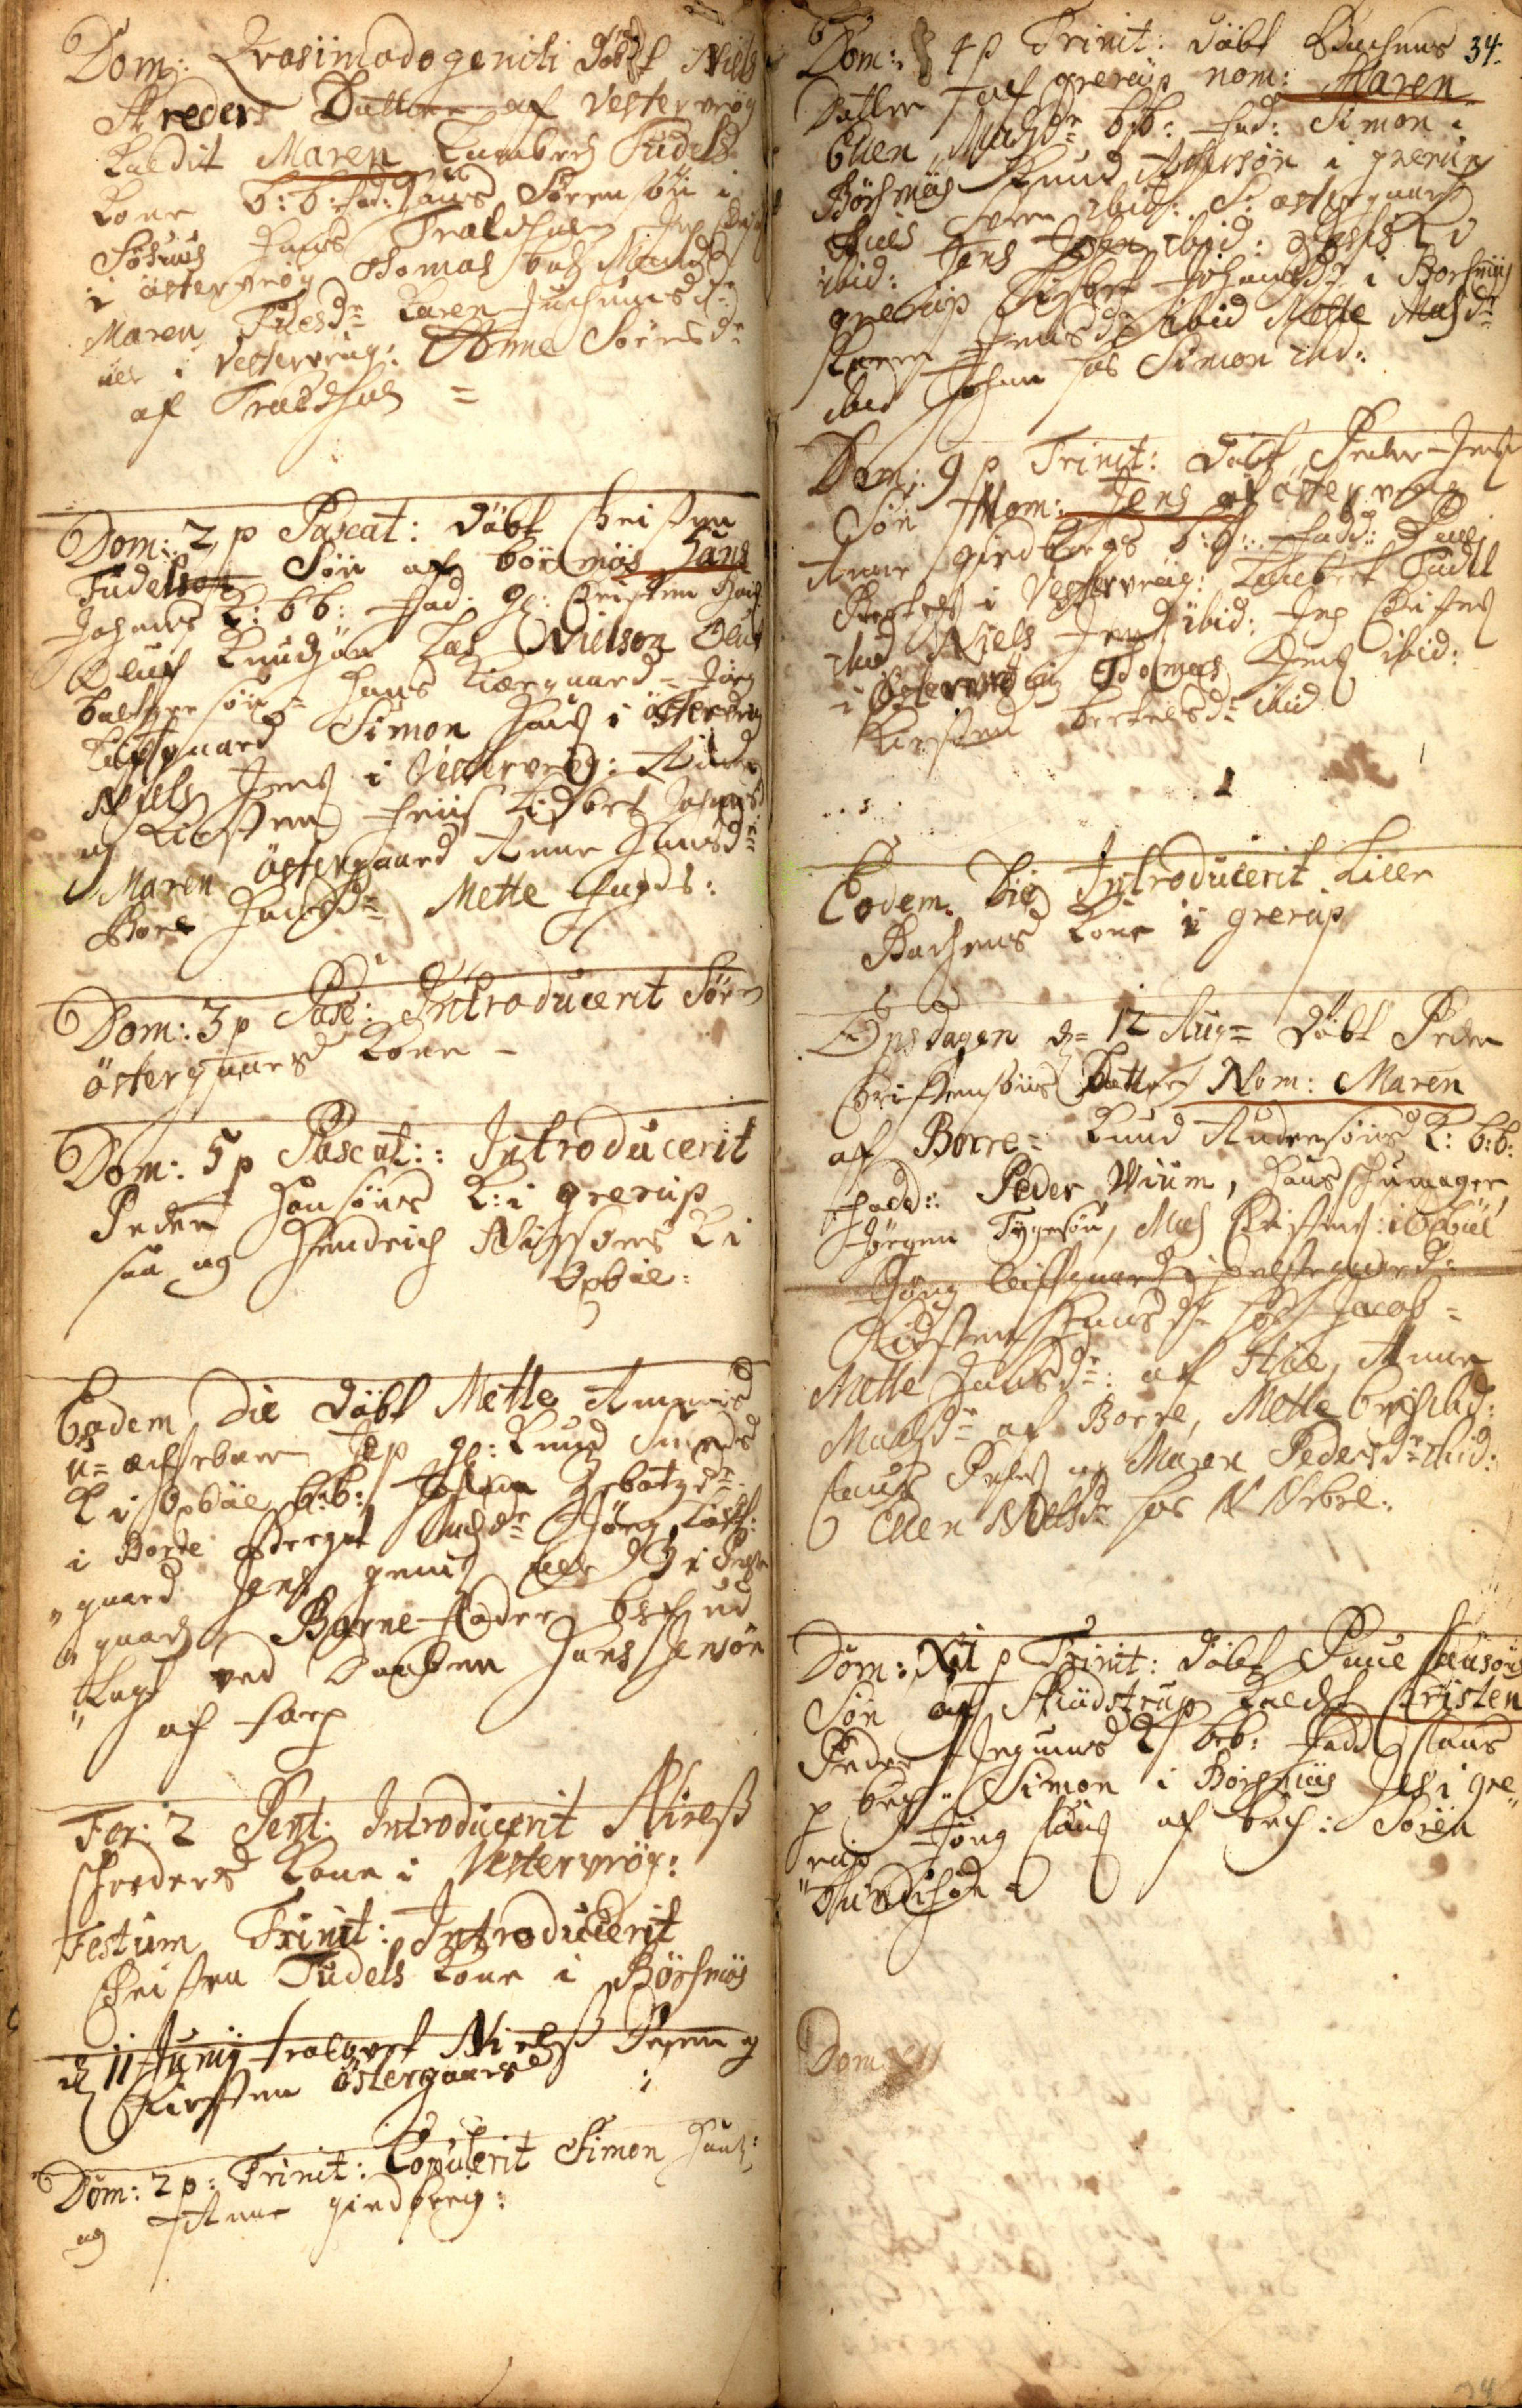Dom: Qvasimodogeniti Døbt Niels
Skreders Datter af Vestervrøg
kaldit Maren Lambert Tudels
Kone b:b: Fad: Hans Sørensøn i
Søhuus Hans Troldholm Jep Christens
i Østervrøg Thomas Bodz Mand
Maren Tuesdr Karen Juchumsdr
## i Vestervrøg: Anne Sørensdr
af Troldhol=
Dom:2 p Pascat: døbt Christen
Tudels Søn af Børsmøs Hans
Johans K: bb.:Fad: gl: Christen Hans
Oluf Knudzøn Las Nielson Oluf
Baltzersøn= Hans Kiærgaard= Jørg
Løftgaard Simon Hans i Østervrøg
Niels Jens i Vestervrøg: Anne
og Kisten Friis Lisbet Johansdr
Maren Østergaard, Anne Hansdr
Boel Hansdr Mette Fogds##
Dom: 3 p Pasc: Introducerit Søren
Østergaards Kone-
Dom: 5 p Pascat:: Introducerit
Peder Hansøns K: i Grerup
saa og Hendrich Nissøns K i
Oxbøl=
Eodem Die døbt Mette Am##
u=chtebarn Jep gl: Knud Smeds## 
K i Oxbøl b:b: Johan## Zebætzdr
i Borre, Berget ##dr Jørg Løft¬
gaard, Jens Gunis Ell## i Præste
gaard Barne-Fader blef ud
lagt ved Daaben Hans Jensøn
af ##rp
Fer: 2 Pent: Introducerit Niels
Schreders Kone i Vestervrøg:
Festum Trinit: Introducerit
Christen Tudels Kone i Børsmøs
D 11 Junij trolovet Niels Degen og
Kirsten Østergaars
Dom: 2 p: Trinit: Copulerit Simon Hans
og Anne Giedberig:
34
34
Dom: ## 4 p Trinit: Døbt Bachens
Datter af Grerup nom: Maren
Ellen Madzdr b:b: Fad: Simon i
Børsmøs Knud Assersøn i Grerup,
Niels Sørens ibid: S. Østergaard
ibid: Jens Johns## ibid: ##s K i
Grerup Lisbet Johansdr i Børsmøs
Karen Jensdr ibid Mette Madzdr
ibid Johan hos Simon ibd:
Dom: 9 p Trinit: døbt Peder Jens
Søn Nom: Jens af Østervrøg
Anne Giedbergs b:b: Fadd:: Pallj
Bertels i Vestervrøg: Lambert Tudel
ibid Niels Jens ibid: Jep Christens
i Østervrøg Thomas Jens## ibid:
Kirsten Bertelsdr ibid
Eodem Die Introducerit Lille
Bachens Kone i Grerup
Onsdagen d=12 Aug= Døbt Peder
Christensøns Datter Nom: Maren
af Borre= Knud Andersøns K: b:b:
Fadd:: Peder Wium, Hans Schumager,
Jørgen Tygesøn, Madz Christens: i Oxbøl,
Jørg Liftgaard i Præstegaard:
Kirsten Hansdr hos Jacob=
Mette Hansdr af Hoe, Anne
Madzdr af Borre, Mette Brich ibid:
Claus Peders og Maren Pedersdr ibid:
Ellen Nielsdr hos N Nebel:
Dom; XI p Trinit: Døbt Poul Clausøns
Søn af Skiødstrup kaldet Christen
Peder Jegums K b:b: Fadd Claus
p Brich" Simon i Børsmøs Jes i Gre¬
rup, Jørg Claus af Brish: Søren
Bundisøn=
Dom